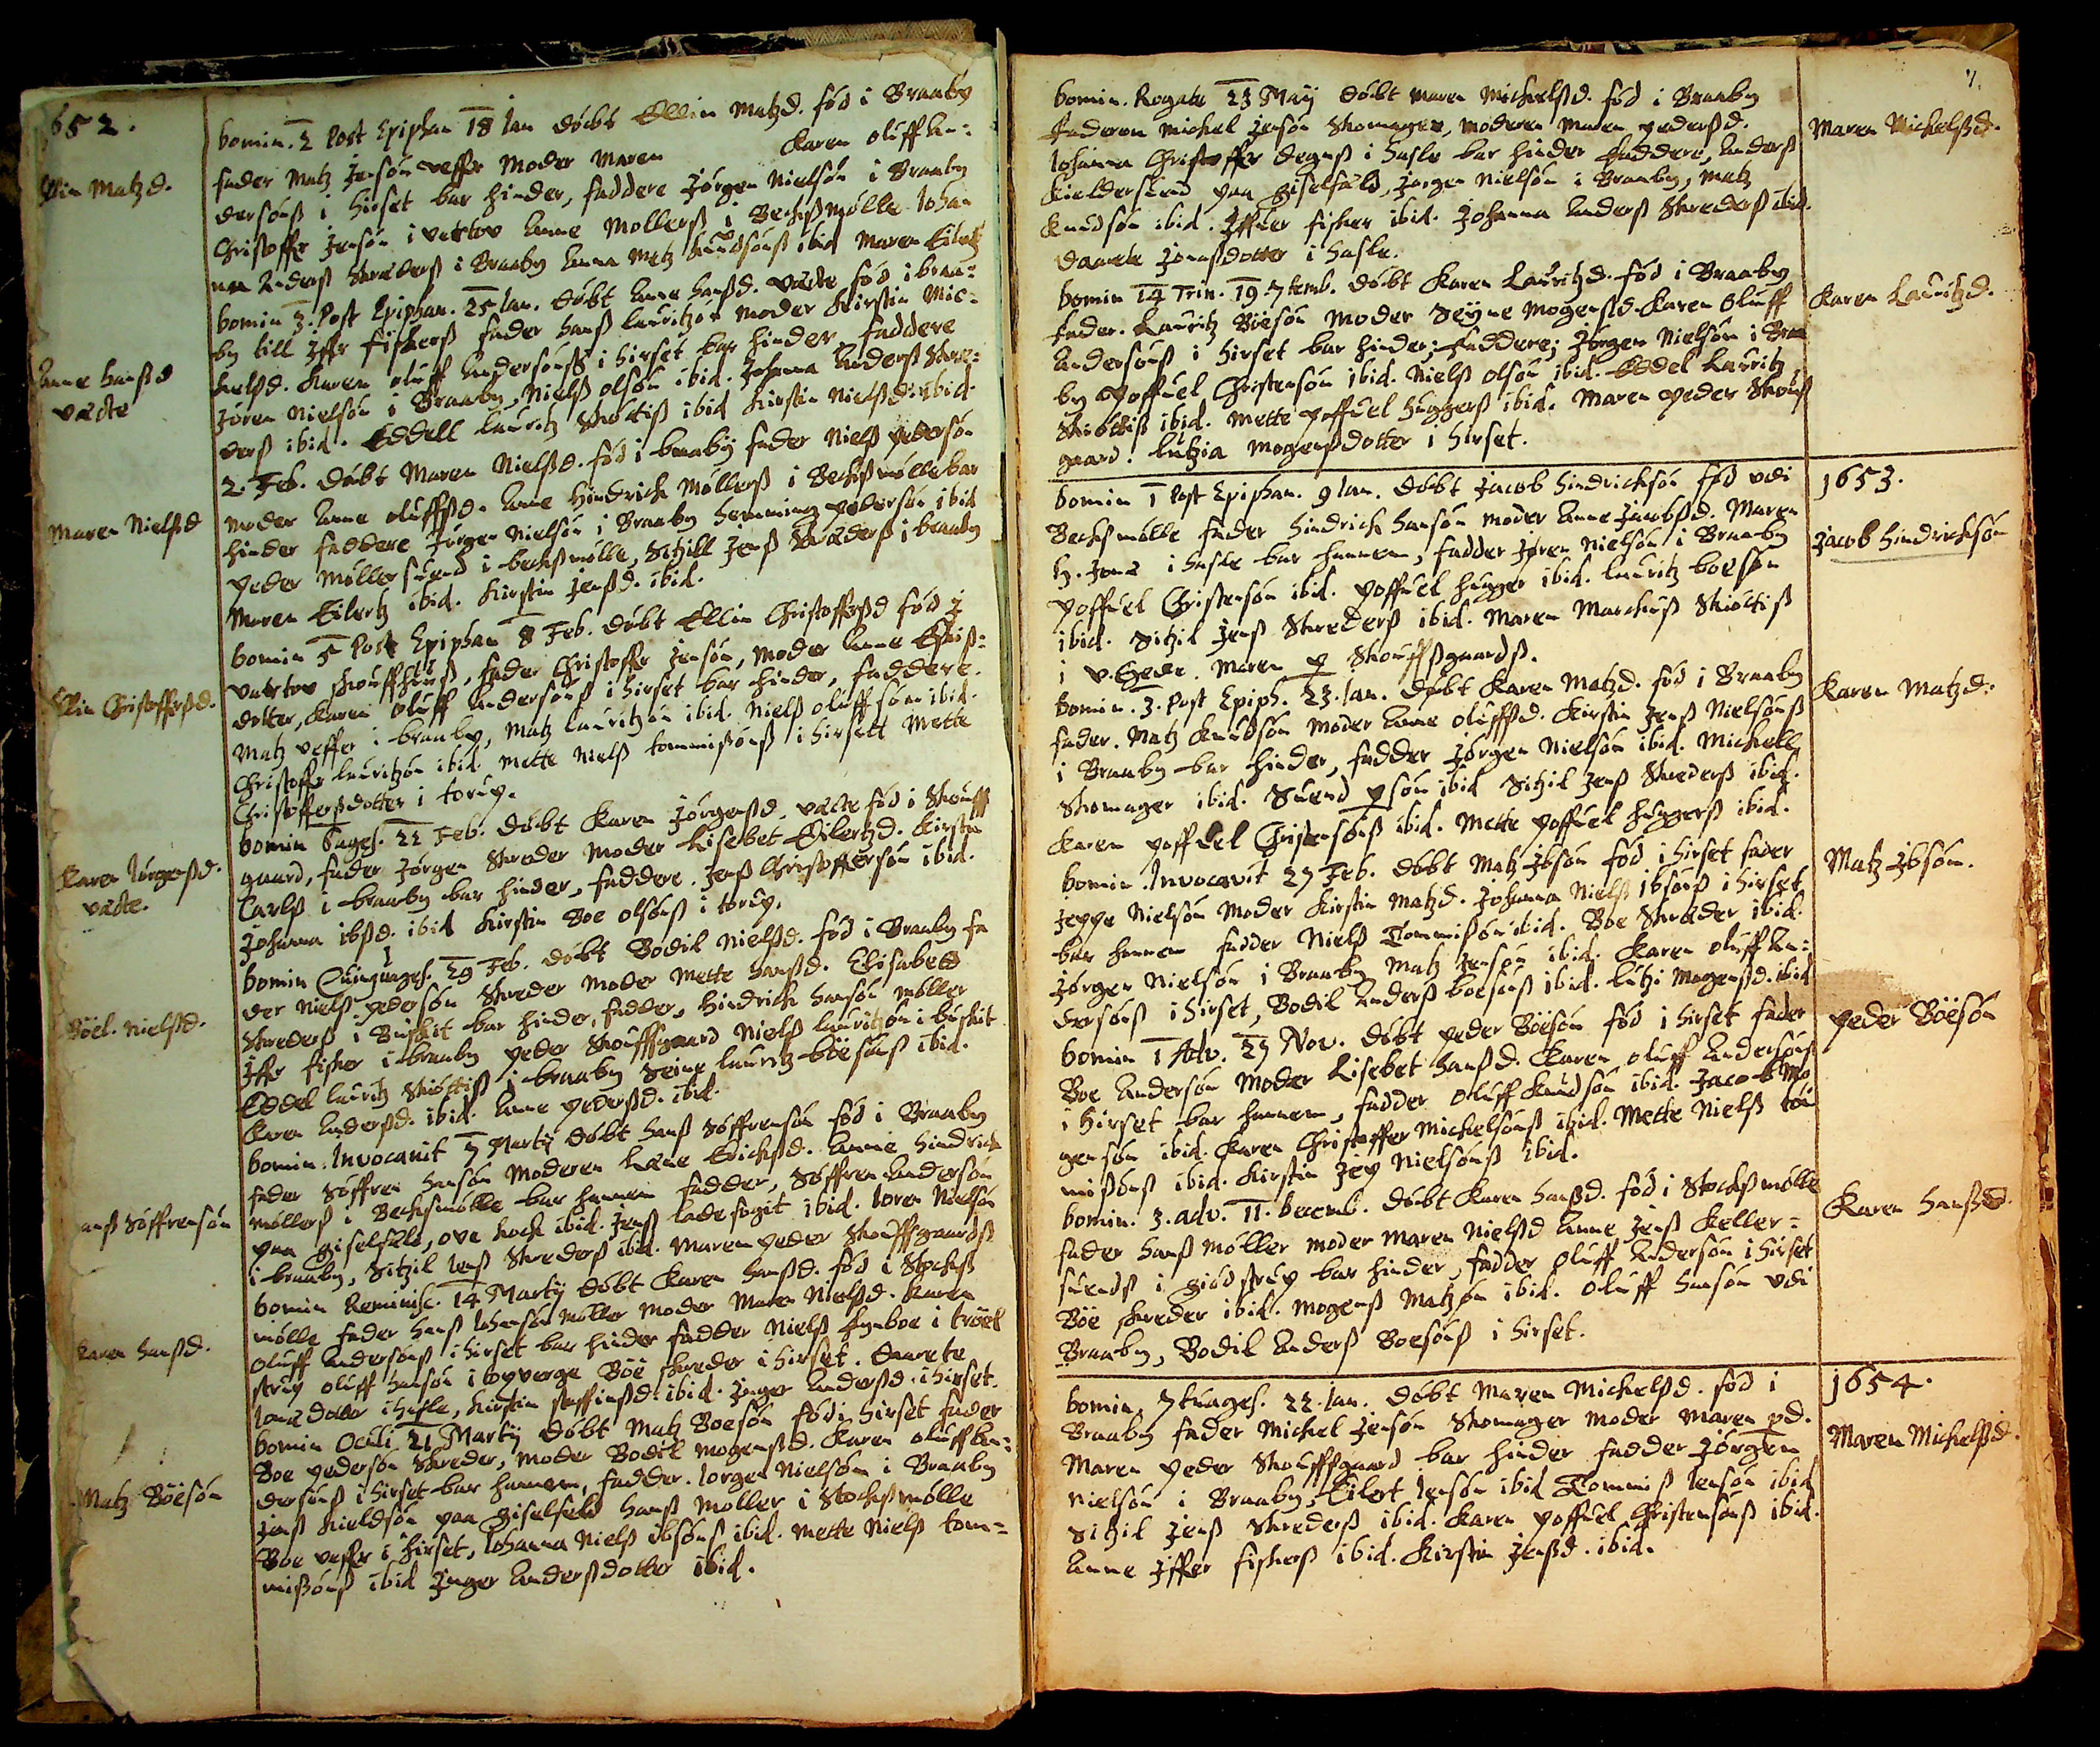652.
Ellin Matzd.
Domin. 2 Post Epiphan 18 Jan døbt Ellin Matzd. fød i Braaby
Fader Matz Jensøn Veffer Moder Maren
Karen Oluff An¬
dersøns i Hirset bar hinder, Faddere Jørgen Nielsøn i Braaby
Christoffer Jensøn i Vartov Anne Møllers i Becksmølle, Joha¬
na Anders Skræders i Braaby Anna Matz Knudsøns ibid. Maren Eilat##
Anne Hansd.
Uæcte
Domin 3. Post Epiphan. 25. Jan. døbt Anne Hansd. Uæcte fød i Braa¬
by till Jffr Fiskers Fader Hans Lauritzøn Moder Kirstin Mic¬
kelsd. Karen Oluff Andersøns i Hirset bar hinder, Faddere
Jøren Nielsøn i Braaby, Niels Olsøn ibid. Johanna Anders Skre¬
ders ibid. Eddill Lauritz Skøetis ibid Kirstin Nielsd. ibid.
Maren Nielsd.
2. Feb. døbt Maren Nielsd. fød i Braaby Fader Niels Pedersøn
Moder Anne Oluffsd. Anne Hendrick Møllers i Beckmølle bar
hinder Faddere Jørgen Nielsøn i Braaby Hemming Pedersøn ibid
Peder Møllersuend i Becksmølle, Sitzill Jens Skræders i Braaby
Maren Eilertz ibid. Kirstin Jensd. ibid.
Ellin Christoffersd.
Domin 5 Post Epiphan 8 Feb. døbt Ellin Christoffersd. fød J
Vartou Skouffhuus, Fader Christoffer Jensøn, Moder Anne Eskis¬
dotter, Karen Oluff Andersøns i Hirset bar hinder, Faddere.
Matz Veffer i Braaby, Matz Lauritzøn ibid. Niels Oluffsøn ibid.
Christoffer Lauritzøn ibid. Mette Niels tommisøns i Hirsett Mette
Christoffersdotter i torup.
Karen Jørgensd.
uæckte
Domin 6ages. 22 Feb. døbt Karen Jørgensd. uæcte fød i Skouss
gaard, Fader Jørgen Skræder, Moder Lisebet Eilartzd. Kirstin
Carls i Braaby bar hinder, Faddere. Jens Christoffersøn ibid.
Johanna ibsd. ibid. Kirstin Boe Olsøns i torup.
Boel. Nielsd.
Domin Quinquages. 29 Feb. døbt Bodil Nielsd. fød i Braaby Fa
der Niels Pedersøn Skreder Moder Mette Hansd. Elisabets
Skreders i Buskit bar hinder, Fadder, Hindrick Hansøn Møller
Jffer Fisker i Braaby Peder Skouffsgaard, Niels Lauritzøn i Buskit
Eddel Lauritz Skiøttis i Braaby Seine Lauritz boesøns ibid.
Karen Andersd. ibid. Anne Pedersd. ibid.
ans Søffrensøn
Domin. Invocavit 7. Marty døbt Hans Søffrensøn fød i Braaby
Fader Søffren Hansøn Moderen Læne Ericksd. Anne Hindrick
Møllers i Becksmølle bar hannem Fadder, Søffren Andersøn
paa Giselfæld, Ove Kock ibid. Jens Ladefogit ibid. Lars Nielsøn
i Braaby, Sitzil Jens Skreders ibid. Maren Peder Skouffsgaards.
Karen Hansd.
Domin Reminisc. 14. Marty døbt Karen Hansd. fød i Stocks
mølle. Fader Hans Johansøn Møller Moder Maren Nielsd. Karen
Oluff Andersøns i Hirset bar hinder Fadder Niels Fynboe i Troel
strup Oluff Hansøn i Toxværge. Boe Skreder i Hirset. Daarete
Larsdotter i Hasle, Kirstin Steffinsd: ibid. Inger Andersd. i Hirset.
Matz Boesøn 
Domin Oculi 21. Marty døbt Matz Boesøn fød i Hirset. Fader
Boe Pedersøn Skreder, Moder Bodil Mogensd. Karen Oluff An¬
dersøns i Hirset bar hannem, Fadder. Jorgen Nielsøn i Braaby
Jens Kildsøn paa Giselfæld, Hans Møller i Stocksmølle
Boe Veffer i Hirset, Johanna Niels ibsøns ibid. Mette Niels tom¬
missøns ibid. Jnger Andersdotter ibid.
Domin. Rogate 23. May døbt Maren Mickelsd. fød i Braaby
Faderen Mickel Jensøn Skomager, Moderen Maren Pedersd.
Johanne Christoffer Degns i Hasle bar hinder. Faddere, Anders
Kieldersvend paa Giselfæld, Jørgen Nielsøn i Braaby, Matz
Knudsøn ibid. Jffuer Fisker ibid. Johanna Anders Skræders ibid.
Daarete Jensdotter i Hasle.
7.
Maren Mickelsd. 
Domin 14 Trin 19. 7temb. døbt Karen Lauritzd. fød i Braaby
Fader: Lauritz Boesøn. Moder Seigne Mogensd. Karen Oluff 
Andersøns i Hirset bar hinder. Faddere; Jørgen Nielsøn i Braa
by Poffuel Christensøn ibid. Niels Olsøn ibid. Eddel Lauritz
Skødis ibid. Mette Poffuel Huggers ibid. Maren Peder Skous
gaard. Lutzia Mogensdotter i Hirset.
Karen Lauristzd.
Domin 1 Post Epiphan. 9. Jan. døbt Jacob Hindricksøn fød udi
Becksmølle Fader Hindrick Hansøn. Moder Anne Jensd. Maren
H. Jonæ i Hasle bar hannem, Fadder: Jøren Nielsøn i Braaby
Poffuel Christensøn ibid. Poffueel hugger ibid. lauritz boesøn
ibid. Sitzil Jens Skreders ibid. Maren Marckus Skiøttis
i V. Egede. Maren p. Skouffsgaards.
1653.
Jacob Hindricksøn
Domin 3. Post Epiph. 23. Jan. døbt Karen Matzd. fød i Braaby
Fader Matz Knudsøn. Moder Anne Oluffsd. Kirstin Jens Nielsøns
i Braaby bar hinder, Fadder Jørgen Nielsøn ibid. Mickell
Skomager ibid. Suend psøn ibid. Sitzil Jens Skreders ibid.
Karen Poffuel Christensøns ibid. Mette Poffuel huggers ibid.
Karen Matzd.
Domin Invocavit 27 Feb. døbt Matz Jbsøn fød i Hirset. Fader
Jeppe Nielsøn Moder Kirstin Matzd. Johanna Niels Ibsøns i Hirset
bar hannem Fadder: Niels Thommisøn ibid. Boe Skredder ibid.
Jørgen Nielsøn i Braaby, Matz Jensøn ibid. Karen Oluff An¬
dersøns i Hirset, Bodil Anders Boesøns ibid. Lutzi Mogensd. ibid
Matz Jbsøn
Domin 1 Adv. 27 Nov. døbt Peder Boesøn fød i Hirset Fader
Boe Andersøn Moder Lisebet Hansd. Karen Oluff Andersøns
i Hirset bar hannem, Fadder Oluff Knudsøn ibid. Jacob Mo
gensøn ibid. Karen Christoffer Mickelsøns ibid. Mette Niels Tø¬
misøns ibid. Kirstin Jep Nielsøns ibid. 
Peder Boesøn
Domin 3. Adv. 11. Decemb. døbt Karen Hansd. fød i Stocksmølle
Fader Hans Møller Moder Maren Nielsd. Anne Jens Keller¬
suends i Giødstrup bar hinder, Fadder Oluff Andersøn i Hirset
Boe Skreder ibid. Mogens Matzøn ibid. Oluff Hansøn udi
Braaby, Bodil Anders Boesøns i Hirset.
Karen Hansd. 
Domin 7 Tuages. 22. Jan. døbt Maren Mickelsd. fød i
Braaby Fader Mickel Jensøn Skomager Moder Maren pd.
Maren Peder Skouffsgaard bar hinder Fadder Jørgen
Nielsøn i Braaby, Eilert Jensøn ibid. Tommis Jensøn ibid
Sitzil Jens Skreders ibid. Karen Poffuel Christensøns ibid.
Anne Jffer Fiskers ibid. Kirstin Jensd. ibid.
1654 
Maren Mickelsd.